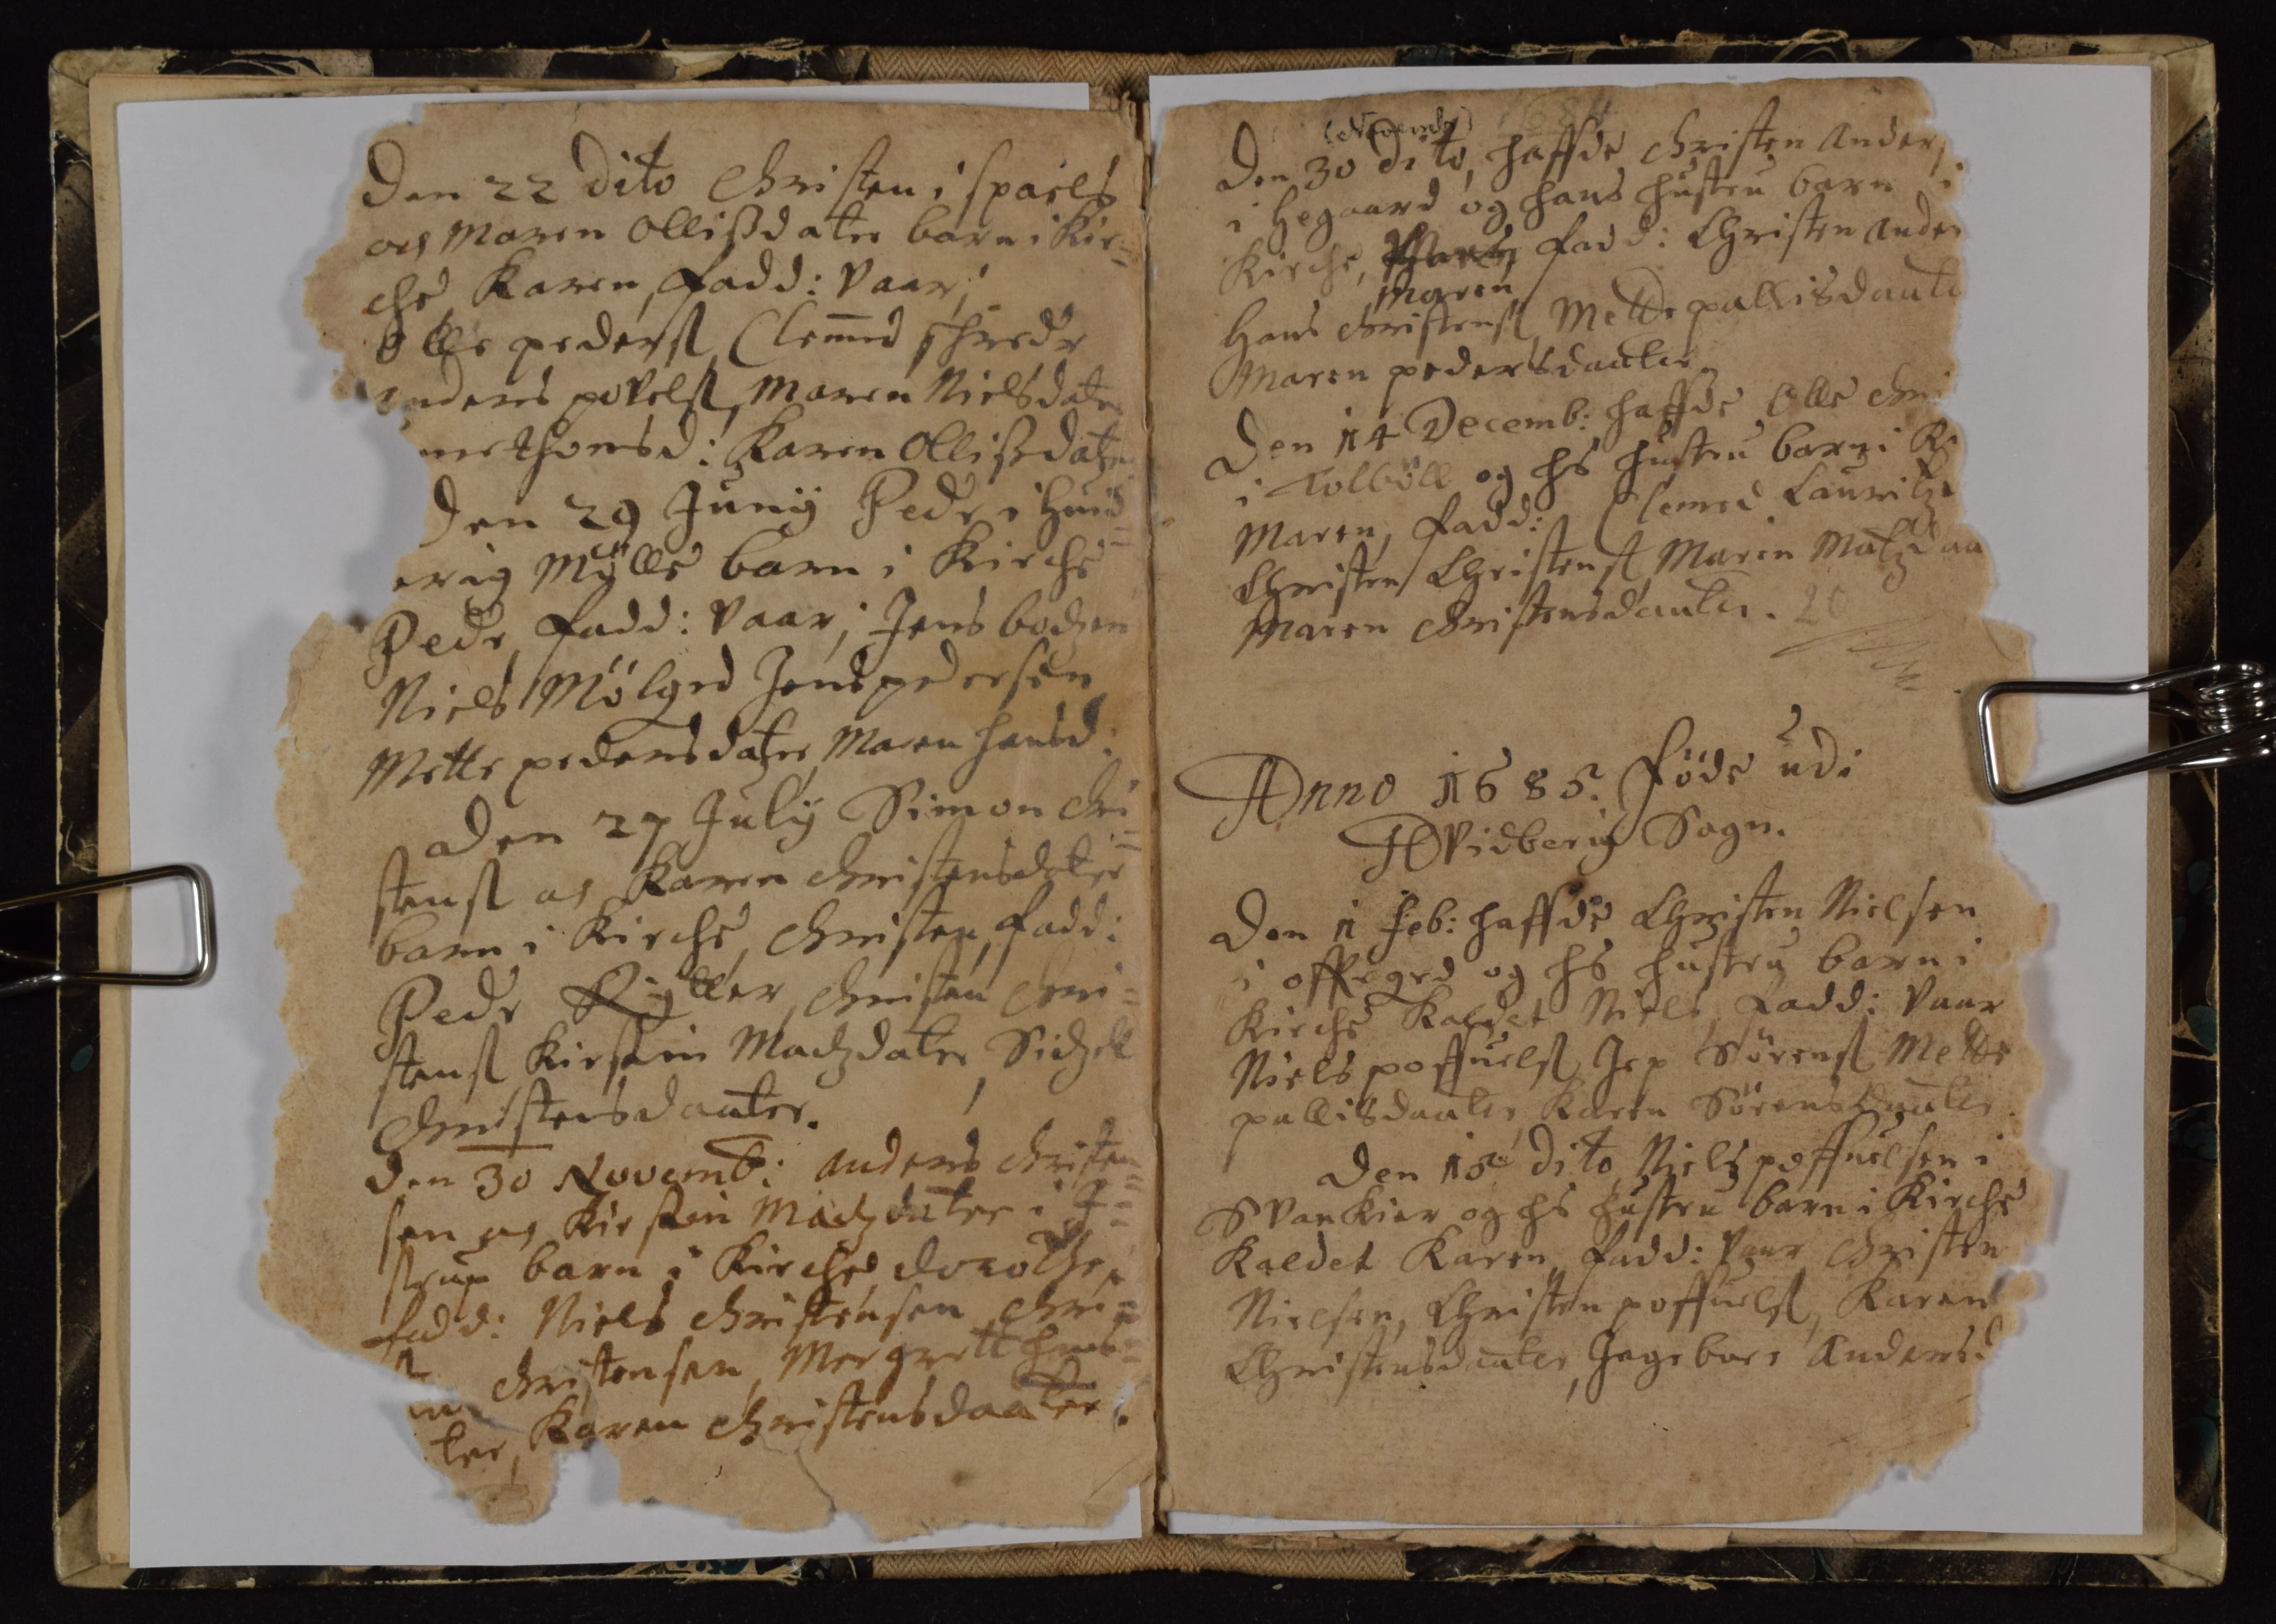Den 22 dito Christen i Spaels
och Maren Ollisdater barn i Kir¬
che, Karen, Fadd: vaar;
Olle peders Clemmed shredr
 nders opvels, Maren Nielsdater
 ne thomsd: Karen Ollissdater
Den 29 Junij Peder i Huid¬
erig Mølle barn i Kirche
Pedr, Fadd: vaar; Jens boden
Niels Mølgrd Jens pedersen
Mette pedersdater Maren hansd:
den 27 Julij Simon Chri¬
stens och Karen Christensdater
barn i Kirche, Christen, Fadd:
Pedr Rytter Chiesten Chri¬
stens Kirstin Madtzdater Sidzell
Christensdauter.
Den 30 Novemb: Anders Christen¬
sen och Kirstin Madzdatter i I¬
strup barn i Kirche, Dorothe,
Fadd: Niels Christensen chri¬
christensen Mergrett thoms¬
ter Karen Christensdaater
(Novembr)
Den 30 Dito, Haffde Christen Anders
i Hegaard og hans hustru barn i
Kirche, ##, Fadd: Christen Ander
Maren
Hans Christens Mette pallisdaute
Maren pedersdauter
Den 14 Decemb: haffde Olle chri
i Tolbøll og hs Hustru barn i K
Maren, Fadd: Clemed Lauritz
Christen Christens Maren Matzdaa
Maren Christensdauter.
Anno 1685 føde udi
Hvidberig Sogn.
Den 11 Feb: haffde Christen Nielsen
i offrgrd og hs hustru barn i
Kirche kaldet Niels, Fadd: vaar
Niels poffuels Jep Sørens Mette
pallisdautter Karen Sørensdater
Den 15 Dito Niels poffuelssen i
Svanrkier og hs hustru barn i Kirche
kaldet Karen. Fadd: vaar Christen
Nielsøn, Christen Poffuels, Karen
Christensdauter, Ingebore Andersd
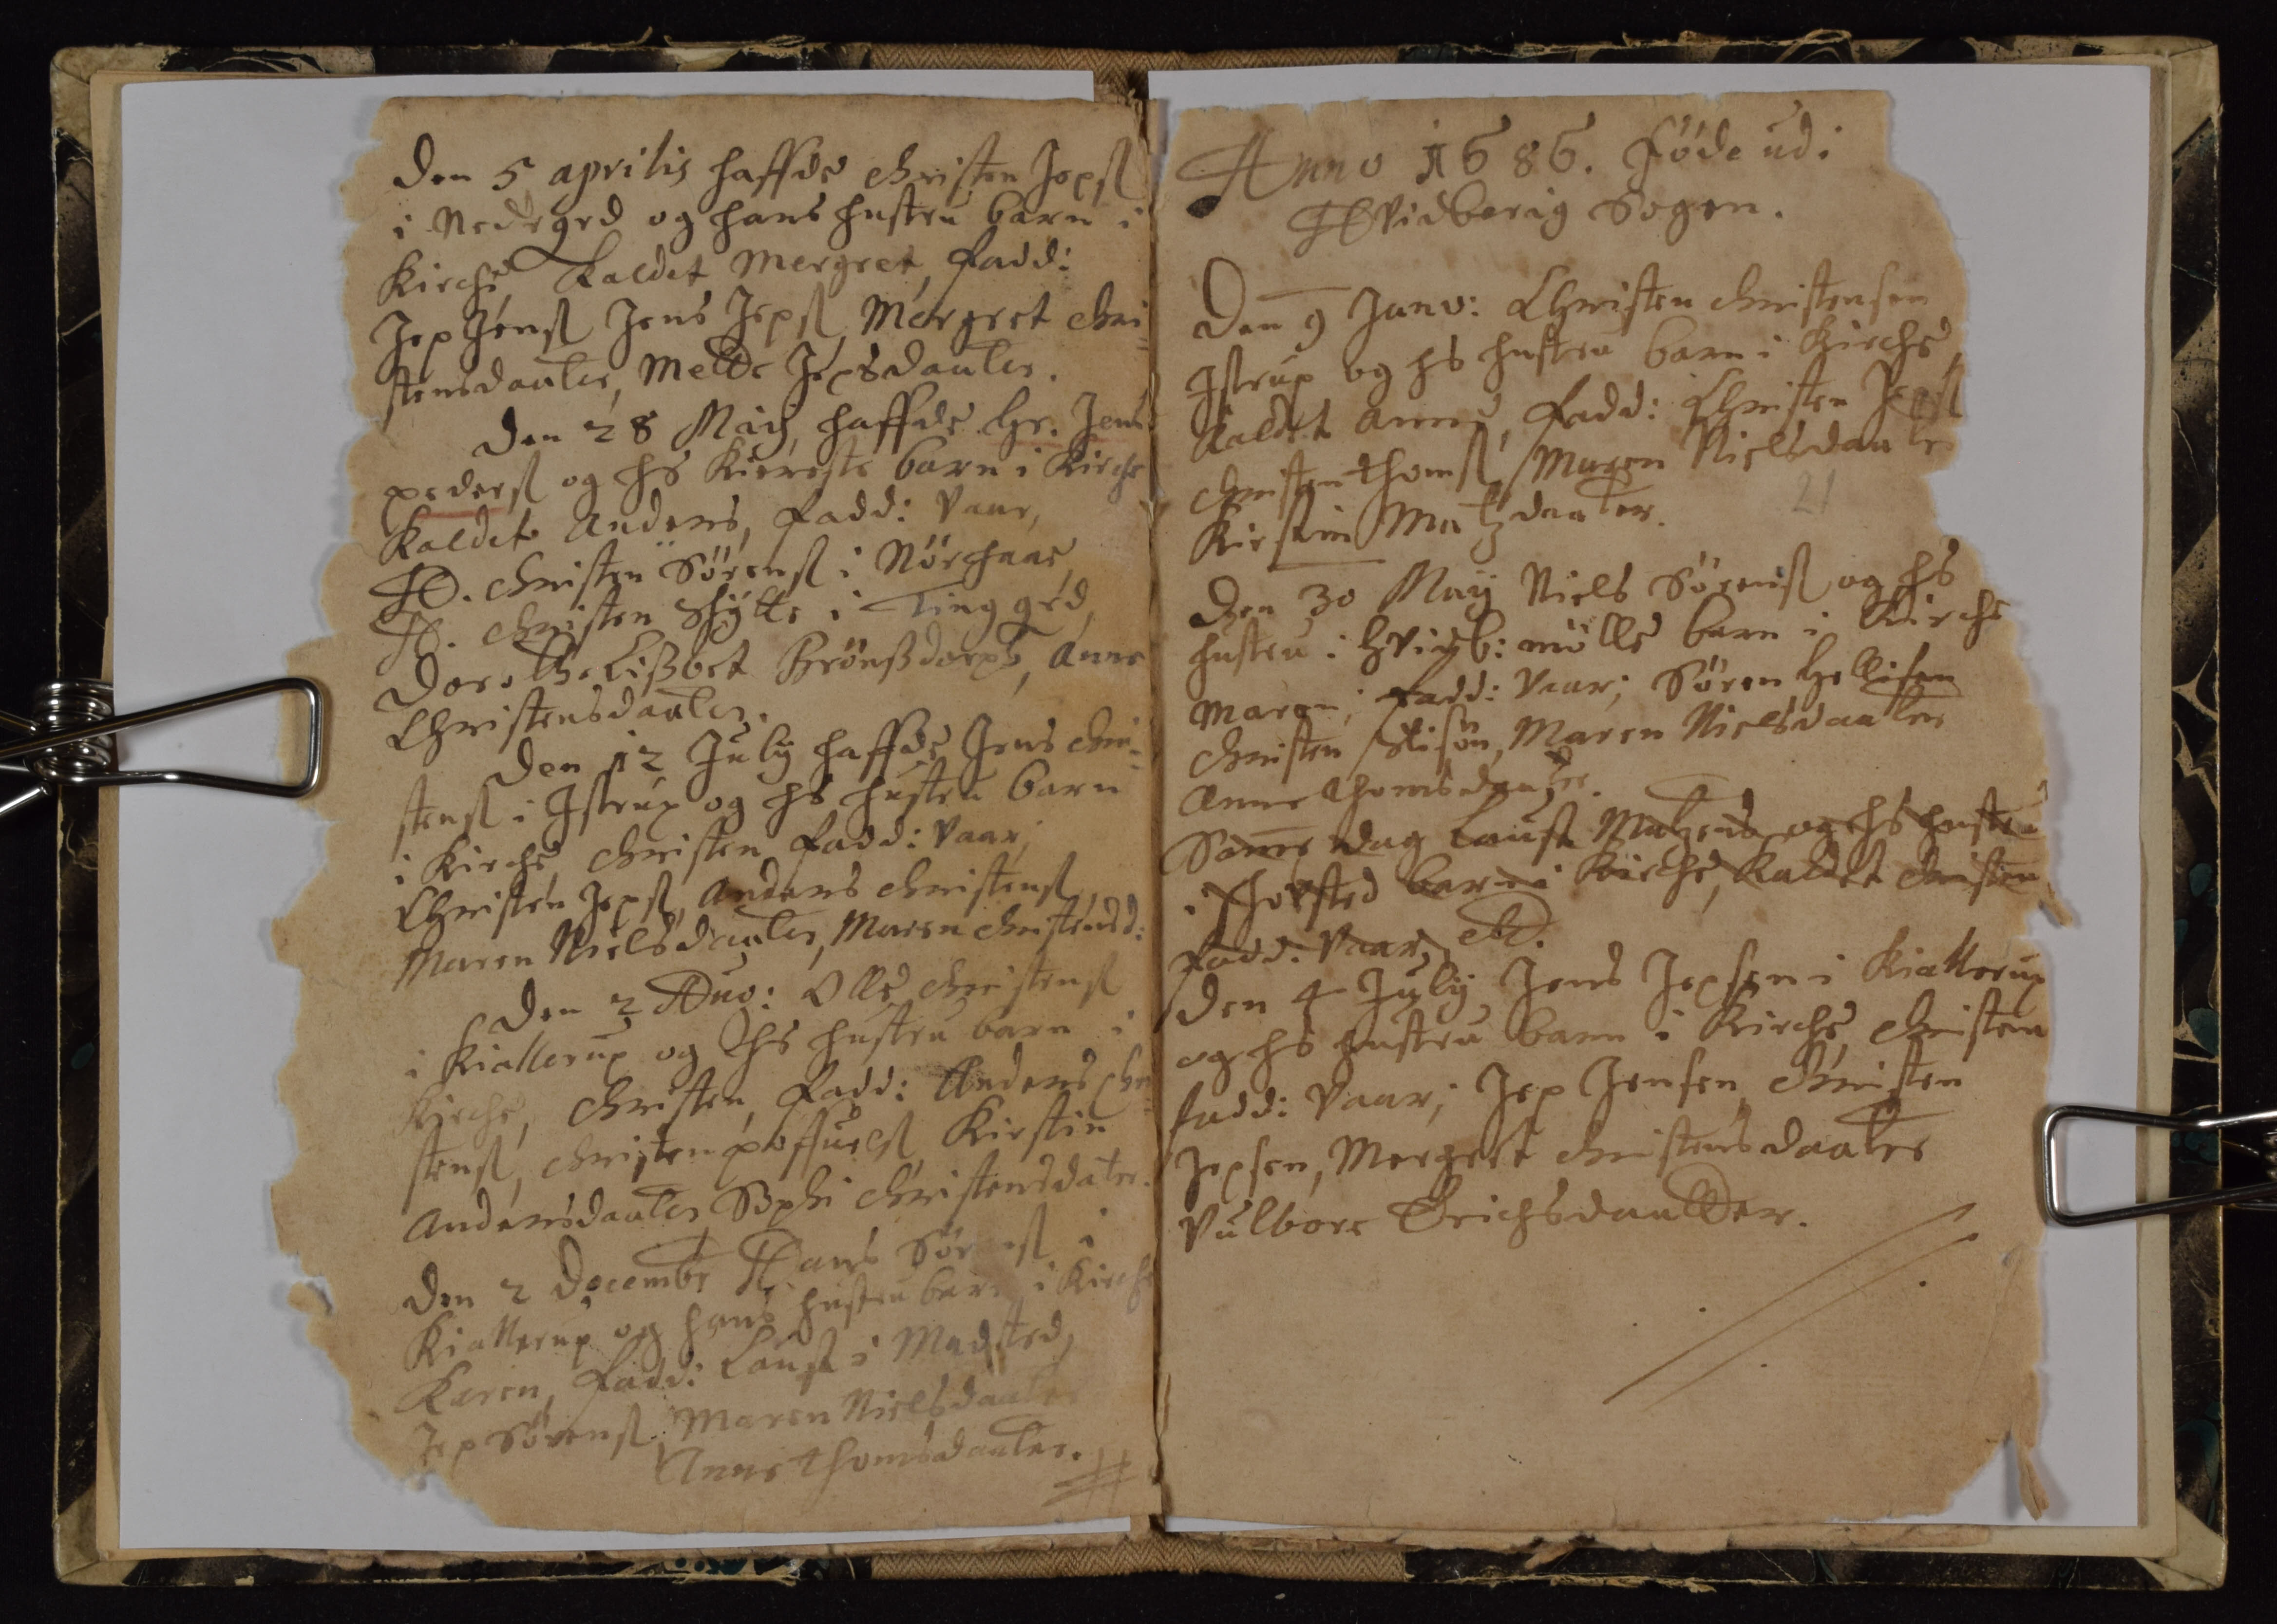Den 5 aprilis haffde Christen Jeps
i Nedrgrd og hans hustru barn i
Kirche, kaldet Mergret, Fadd:
Jep Jens Jens Jeps Mergret Chr¬
stensdauter Mette Jepsdauter
Den 28 Maij, haffde Hr: Jens
Peders og hs Kiereste barn i Kirche
kaldet Anders, Fadd: vaar
H. Christen Sørens i Nørchaae
H.b: Christen Shytte i Tinggrd
Dorothe Lisbet Grønssdorp, Anne
Christensdauter.
Den 12 Julij haffde Jens chri¬
stens i Istrup og hs hustru barn
i Kirche, Christen Fadd: vaar;
Christen Jeps Anders Christens
Maren Nielsdauter, Maren christensd:
den 2 Aug: Olle Christens
i Kiallerup og hs hustru barn i
Kirche, Christen, Fadd: Anders Chri¬
stens, christen poffuels, Kirstin
Andersdauter Sophi Christensdater
Den 2 Decembr Hans Sørens i
Kiallerup og hans hustru barn i Kirche
Karen, Fadd: Laust i Madsted,
Jep Sørens, Maren Nielsdautter
Anne Thomsdauter.
Anno 1686. Føde udi
Hvidberig Sogen.
Den 9 Janv: Christen christensen
Istrup og hs hustru barn i Kirche
kaldet Anne, Fadd. Christen Je##
christen thoms Maren Nielsdauter
Kirstin Matzdaater
Den 30 Maij Niels Søfrens og hs
hustru i Hvidb:: mølle barn i Kirche
Maren; Fadd: vaar; Søren Hellisen
christen Stisøn, Maren Nielsdaater
Anne Thomsdauter.
Samme Dag Laust Matzens og hs hustru
i Shovsted barn i Kirche, kaldet christen
Fadd: vaar etc.
Den 4 Julij Jens Jepsen i Kiallerup
og hs hustru barn i Kirche, christen
Fadd: vaar Jep Jensen christen
Jepsen, Mergret christensdaater
Vulbore Erichsdautter.